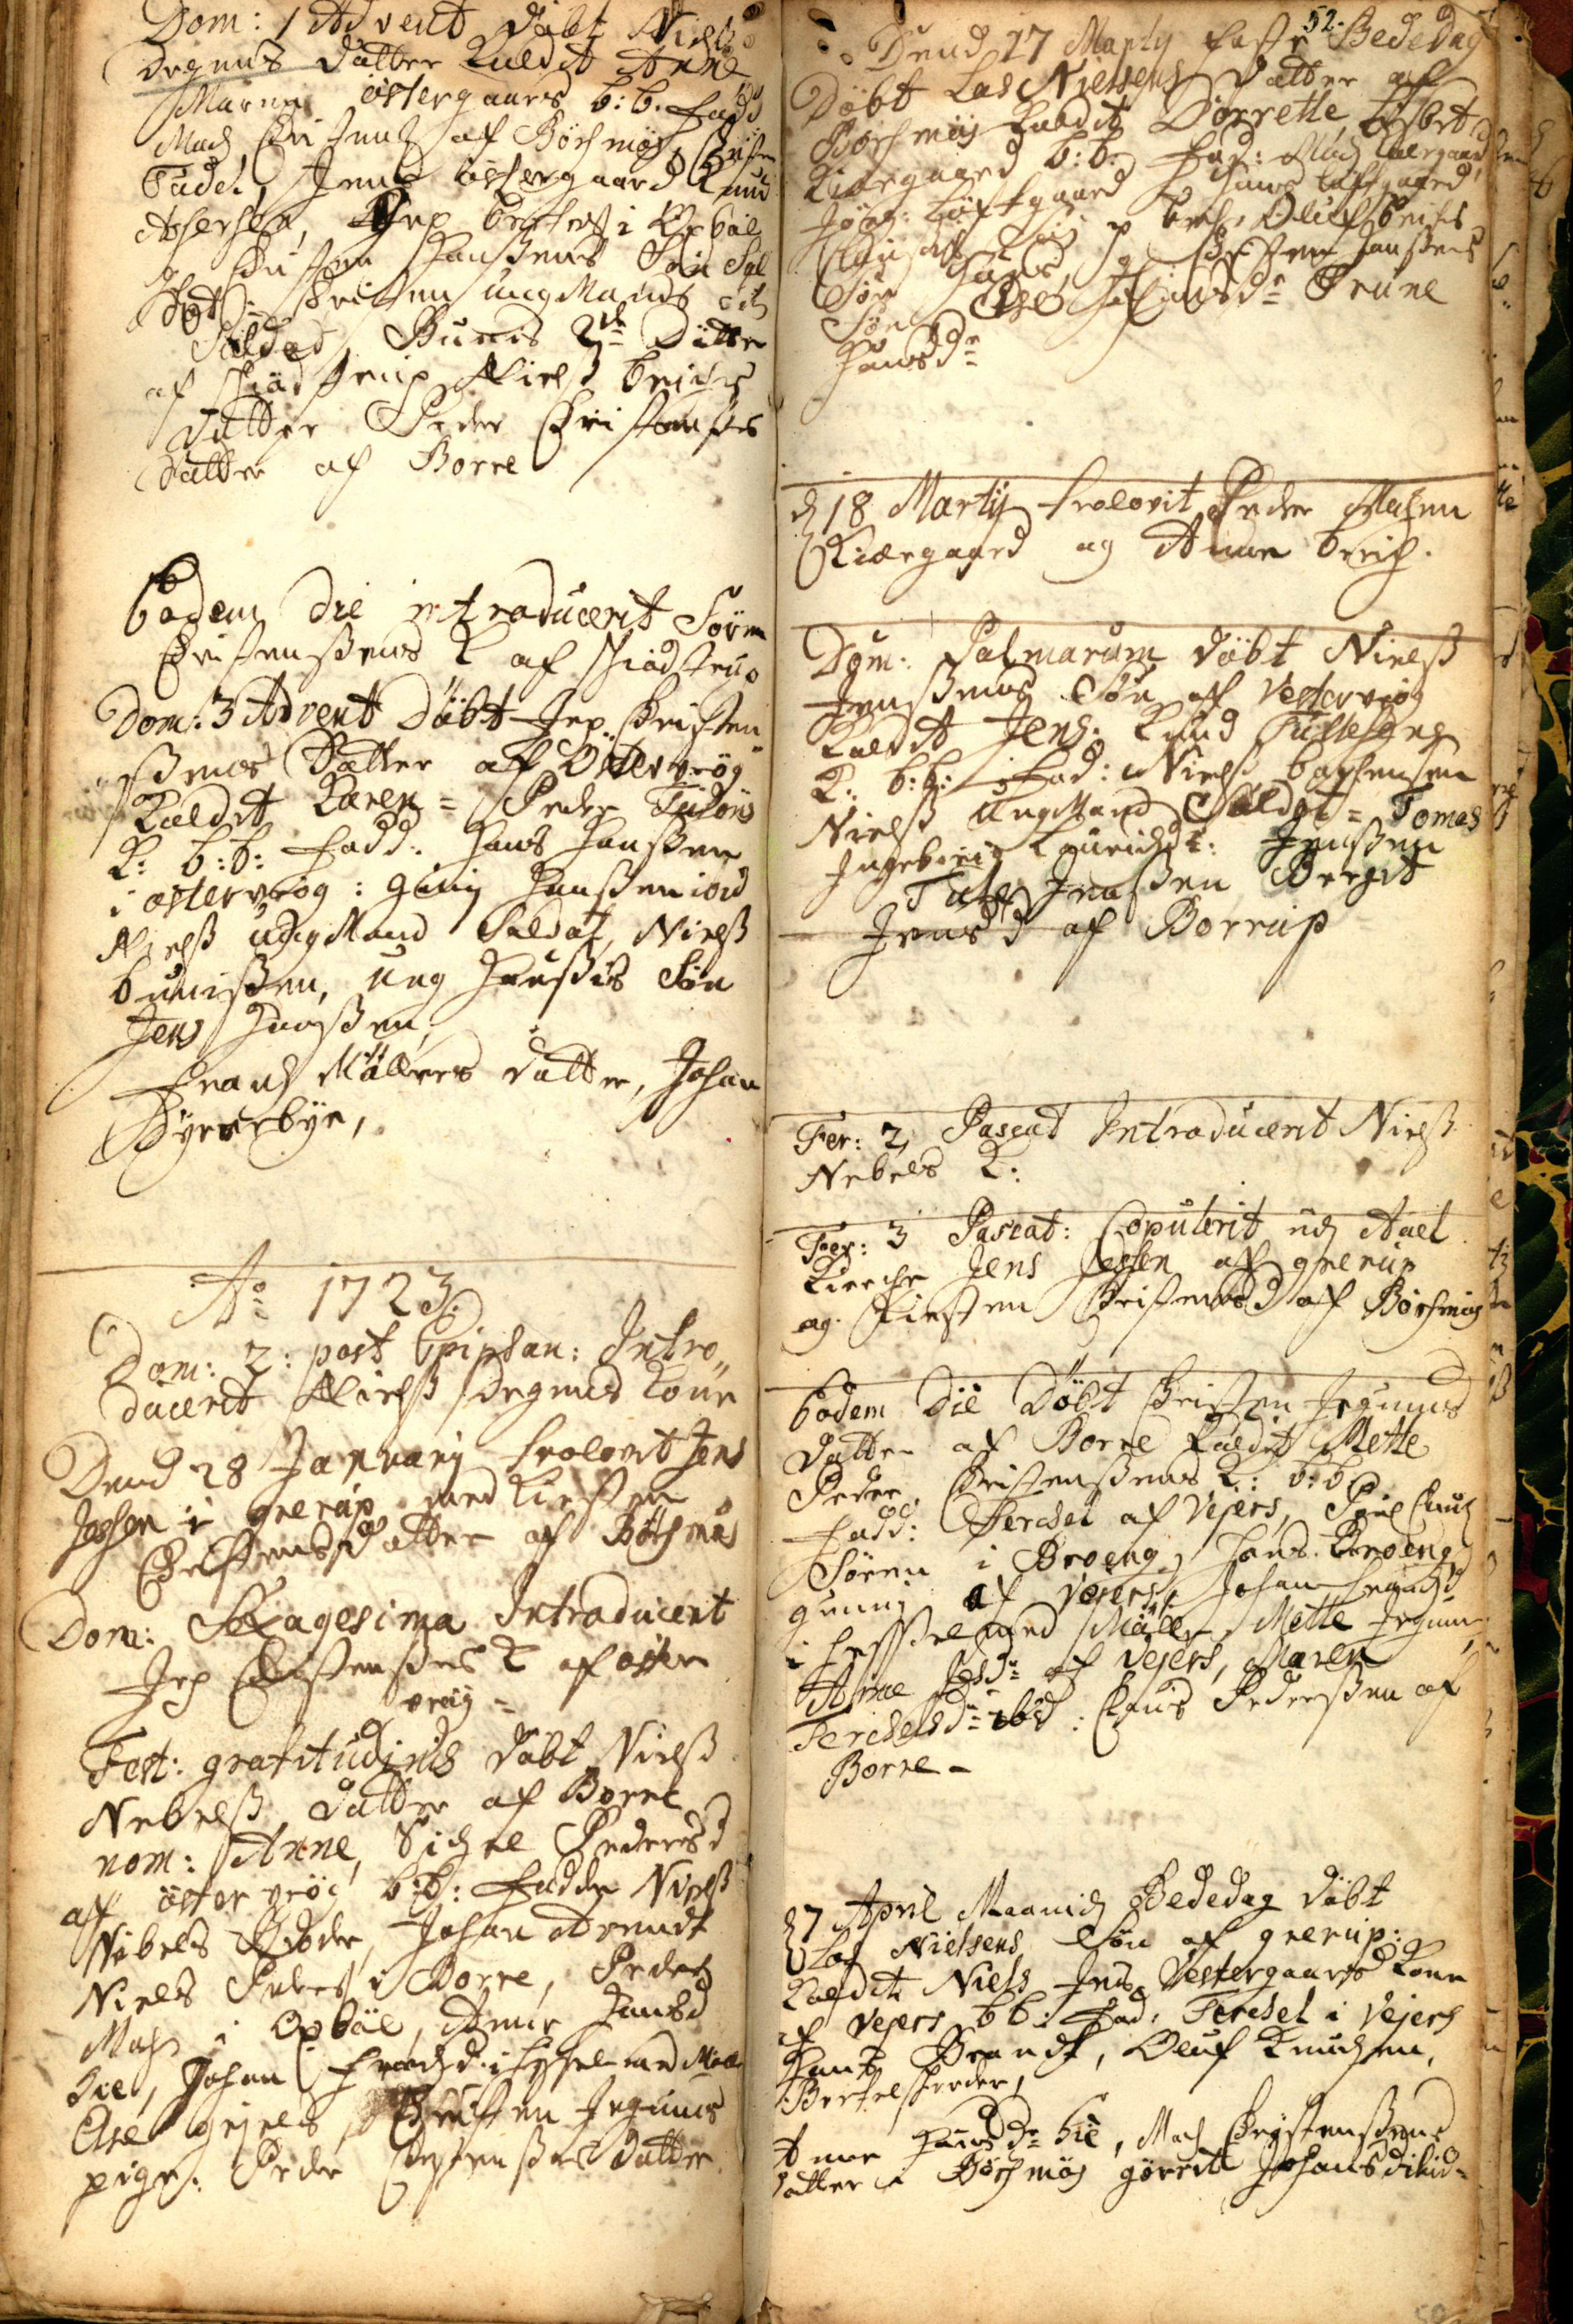Dom: 1 Advent døbt Niels
Degens Datter kaldit Anne
Maren Østergaars b:b: Fadd
Madz Christens af Børsmøs, Christen
Tudel, Jens Østergaard Knud
Assersen, Jep Bertelsen i Oxbøl
gl Christen Hansens Søn Sol
dat= Christen ung Mands dito
Soldat Bunis 2de Døttre
af Schiødstrup, Niels Brichis
Datter Peder Christensens
Datter af Borre
Eodem Die introducerit Søren
Christensens K af Schiødstrup
Dom: 3 Advent Døbt Jep Christen
sens Datter af Østervrøg
kaldit Karen= Peder Tusøns
K: b:b: Fadd: Hans Hansen
i Østrvrøg: Gunj Hansen ibid
Niels ung Mand Soldat, Niels
Bunisen, ung Hansis Søn
Jens Hansen
Frandz Møllers Datter, Johan
Dyrrebye,
Ao 1723.
Dom: 2: post Epiphan: Intro¬
ducerit Niels Degens Kone
Dend 28 Januarij trolovet Jens
Jessen i Grerup med Kirsten
Christensdatter af Børsmøs
Dom: Sexagesima Introducerit
Jep Christensens K af Øster
vrøg=
Fest: Gratitudinis døbt Niels
Nebels Datter af Borre
nom: Anne, Sidzel Pedersd
af Østervrøg b:b: Faddere Niels
Nebels Broder, Johan Arendt
Niels Peders i Borre, Peder
Mads i Oxbøl, Anne Hansd
##, Johanne Frandzd i Hisselmed Mølle
Else Gejels, Christen Jegums
pige: Peder Christensens Datter
52
50
Dend 17 Martij Faste Bededag
Døbt Las Nielsens Datter af
Børsmøs kaldit Dorrette, Lisbet
Kiærgaard b:b: Fad: Madz Kiærgaard
Jørg: Løftgaard Hans Løftgaard
Clauses Søn p Brich, Oluf Brichis
Søn Hans, gl Christen Hansens
Søn Else Johansdr Triine
Hansdr
D 18 Martij trolovit Peder Madzen
Kiærgaard og Anne Brich.
Dom: Palmarum døbt Niels
Jensens Søn af Vestervrøg
kaldit Jens. Knud Tullesens
K: b:b: Fad: Niels Bachensen
Niels ung Mand Soldat= Tomas
Ingeborig Lauridzdr Jensen##
Tule Jensen Bergit
Jensd af Borrup
Fer: 2 Pascat Introducerit Niels
Nebels K:
Fer: 3 Pascat: Copulerit udi Aael
Kirche Jens Jessen af Grerup
og Kirsten Christensd af Børsmøs
Eodem Die Døbt Christen Jegums
Datter af Borre kaldit Mette
Peder Christensens K: b:b:
Fadd: Therchel af Vejers, Poul Claus
Søren i Broeng, Hans Broeng
Gunnj af Vejers, Johanne Frandzdr
i Hisselmed Mølle, Mette Jegum,
Anne Jesdr af Vejers, Maren
Terchelsdr ibid: Claus Pedersen af
Borre
D 7 April Maanedz Bededag døbt
Las Nielsens Søn af Grerup:
kaldit Niels, Jes Vestergaars Kone
af Vejers bb: Fad: Terchel i Vejers,
Hans Brandt, Oluf Knudzen
Bertel Schreder,
Anne Hansdr hic##, Madz Christensens
Datter i Børsmøs Gørrit Johansd ibid:
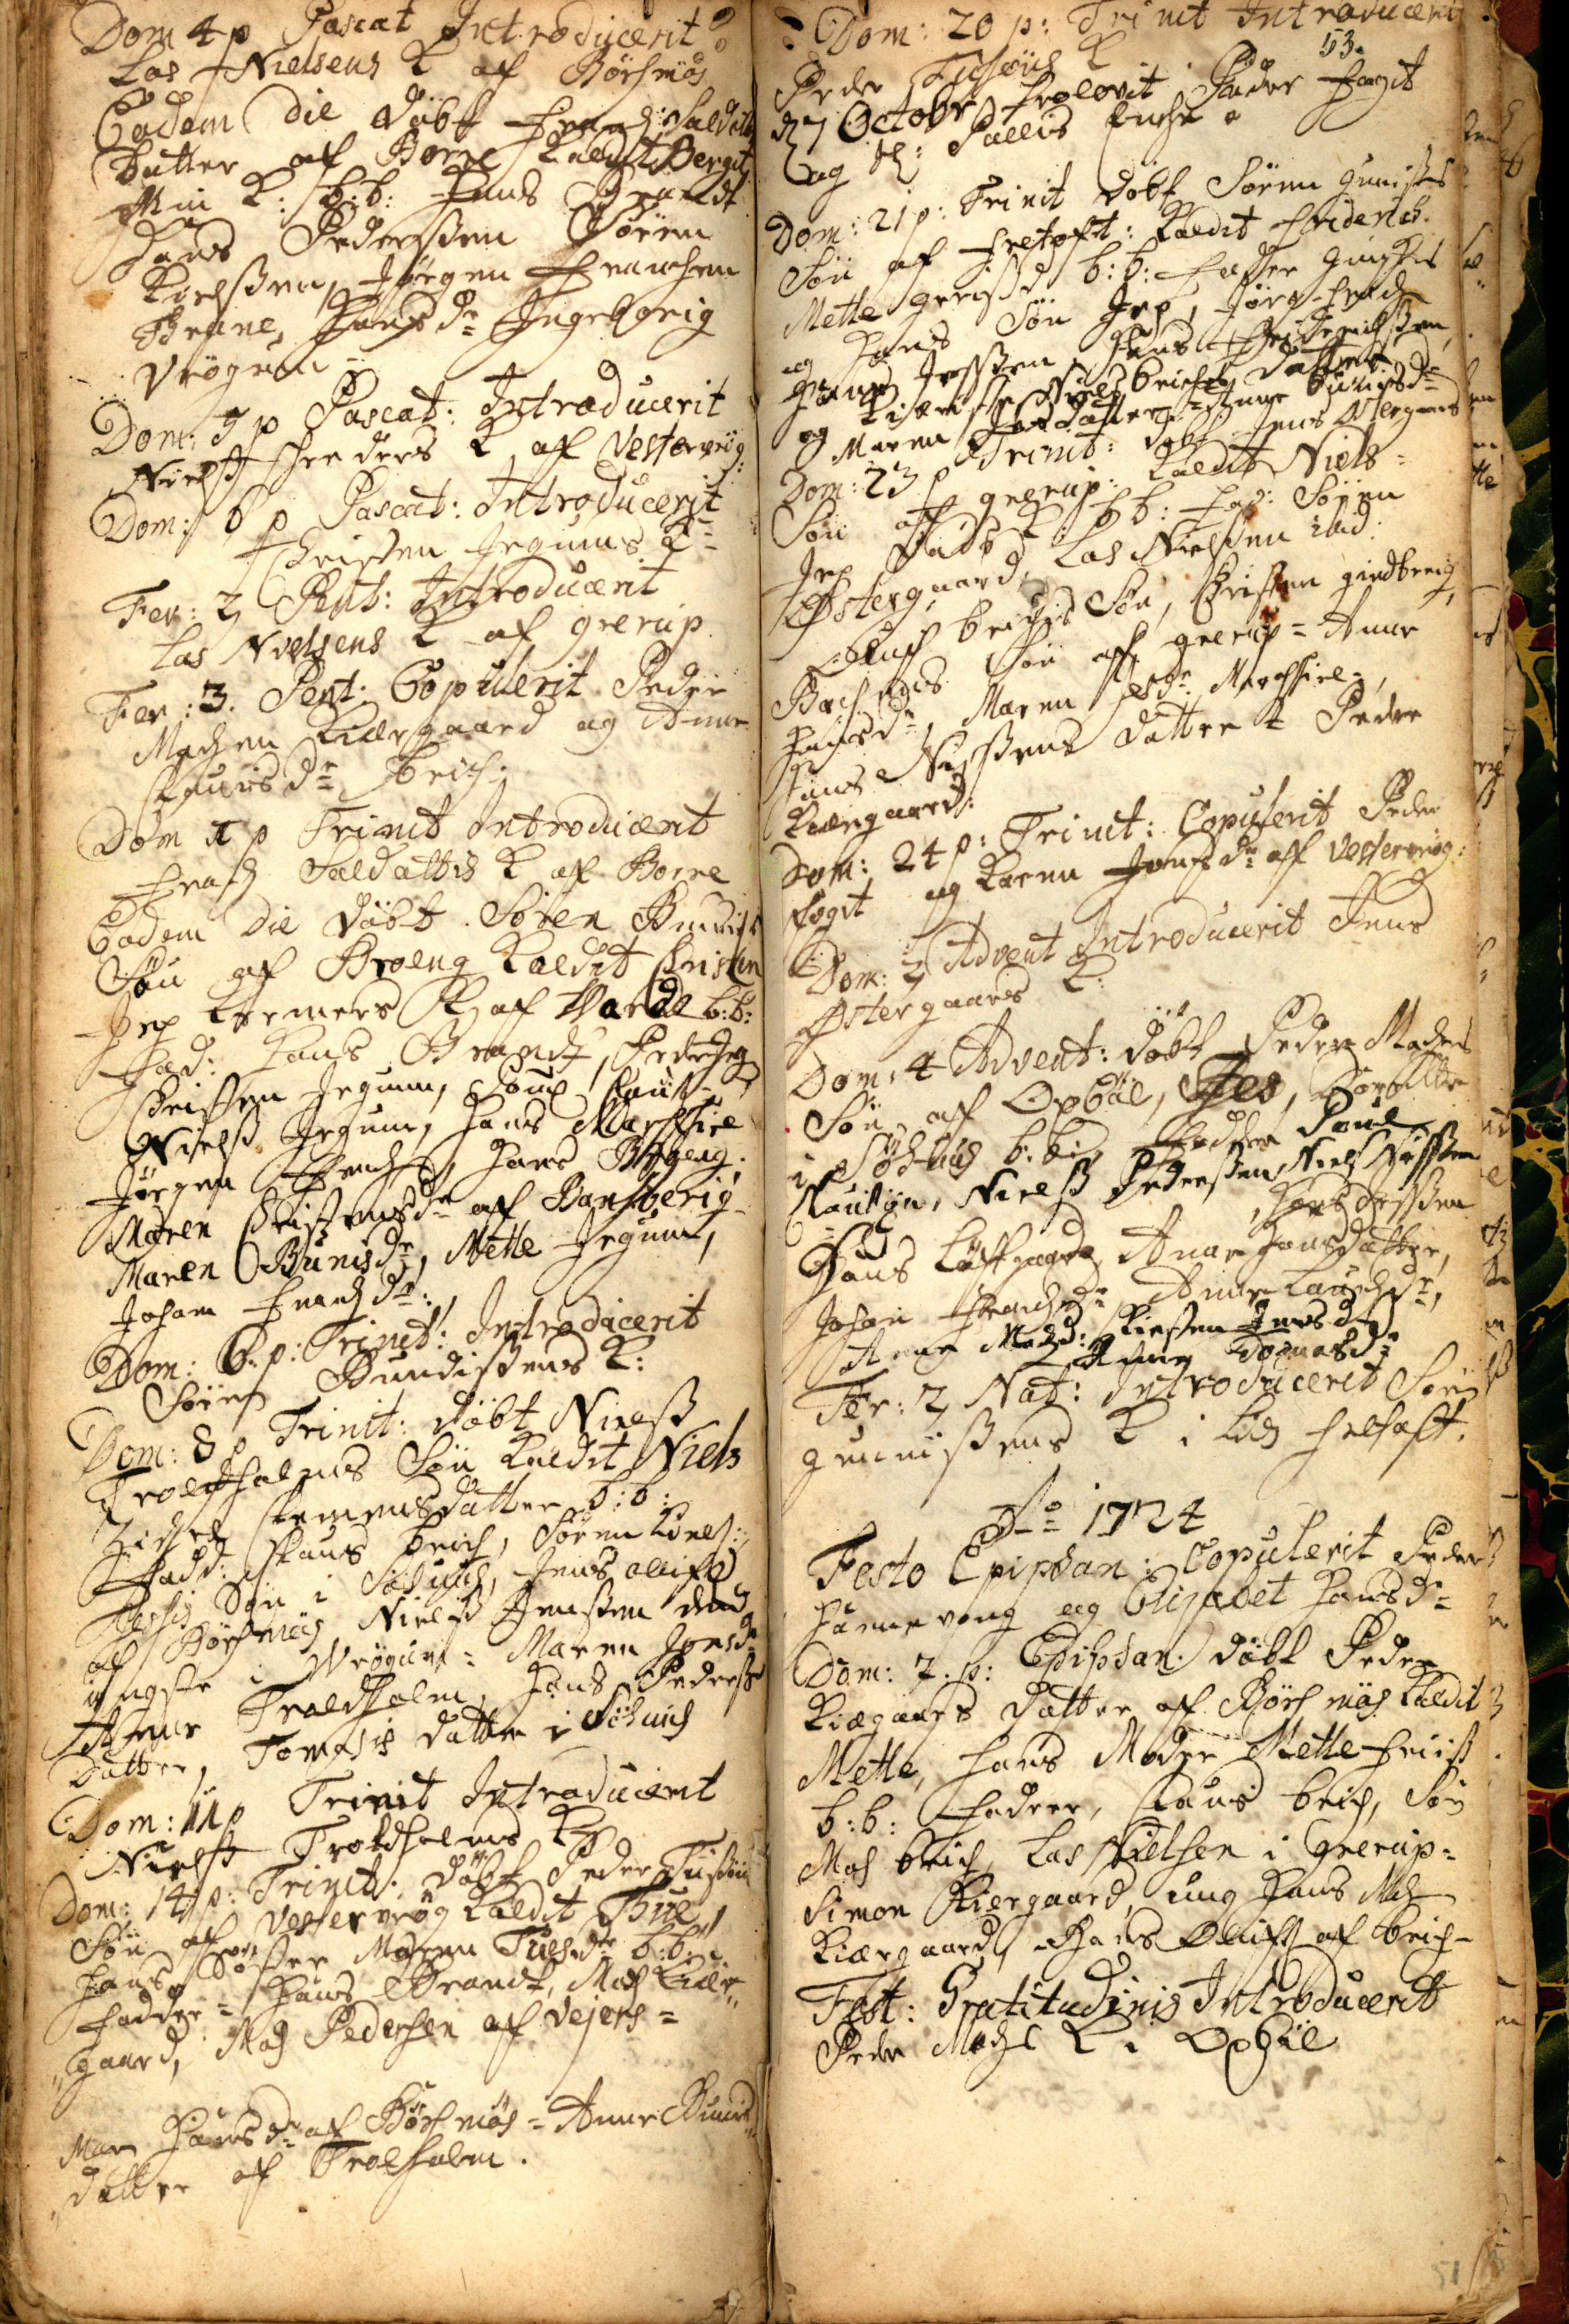Dom 4 p Pascat Introducerit
Las Nielsens K af Børsmøs
Eodem Die døbt Farndz Soldats
Datter af Borre kaldit Bergit
Min K: b:b: Hans Brandt
Hans Pedersen Søren
Kielsen, Jørgen Frandzen
Thriine Hansdr Ingeborig
Vrøgum
Dom: 5 p Pascat: Introducerit
Niels Schreders K af Vestervrøg
Dom: 6 p Pascat: Introducerit
Christen Jegums K=
Fer: 2 Pent: Introducerit
Las Nielsens K af Grerup
Fer: 3. Pent: Copulerit Peder
Madzen Kiærgaard og Anne
Clausdr Brich:
Dom 1 p Trinit Introducerit
Frandz Soldattis K af Borre
Eodem Die døbt Søren Bunnisens
Søn af Broeng kaldit Christen
Jep Kremers K af Warde b:b:
Fad: Hans Brandt, Peder Jeg
Christen Jegum, Poul Claus=
Niels Jegum, Hans Marchschiel
Jørgen Christens, Hans Broeng
Maren Christensdr af Bansberig
Maren Bunisdr, Mette Jegum,
Johanne Frandzdr:
Dom: 6: p: Trinit: Introducerit
Søren Bundisens K:
Dom: 8 p Trinit: døbt Niels
Troldholms Søn kaldit Niels
Zidzel Clemensdatter b:b:
Fadd: Claus Brich, Søren Kiels:
Jessis Søn i Søhuus, Jens Olufs
af Børsmos Niels Jensen dend
yngste i Wrøgum: Maren Jonsdr
Anne Troldholm, Hans Peders
Datter Tomasis Datter i Søhuus
Dom: 11 p Trinit Introducerit
Niels Troldholms K
Dom: 14 p: Trinit: døbt Peder Tussøns
Søn af Vestervrøg kaldit Thue,
Hans Søster Maren Tuesdr b:b:
Fadder= Hans Brandt, Madz Kiær¬
gaard, Madz Pedersen af Vejers=
Maren Hansdr af Børsmøs= Anne Bundis¬
datter af Troldholm.
53
51
Dom: 20 p:Trinit Introducerit
Peder Tusøns K.
D 7 Octobr trolovet Peder Foget
og Sl Pallis Enche ##
Dom: 21 p: Trinit døbt Søren Gunisens
Søn af Fretoft: kaldit Friderich.
Mette Greisd b:b:Fadder Gunj H##
og hans Søn Jep, Jørgen Frandz
Hans Jessen, Hans Friderichsen
og Kiæreste, Niels Brichis Datter.
Maren Jesdatter= Anne Tullisdr
Dom: 23 p Trinit: døbt Jens Østergaars
Søn af Grerup: kaldit Niels=
Jep Vads K: bb: Fad: Søren
Østergaard, Las Nielsen ibid:
Oluf Brichis Søn, Christen Giedberig
Bachens Søn af Grerup= Anne
Hansdr, Maren Jesdr Marchschiel=
Hans Nissens Datter=  Peder
Kiærgaard:
Dom: 24 p: Trinit: Copulerit Peder
Fogit og Karen Jonsdr af Vestervrøg:
Dom: 2 Advent Introducerit Jens
Østergaars K:
Dom: 4 Advent: døbt Peder Madzens
Søn af Oxbøl, Jes, Dorrette
i Søhuus b:b: Faddere Poul
Clausøn, Niels Pedersen, Niels Nissen
Hans Jessen
Hans Løftgaard, Anne Hansdatter,
Johan Frandzdr Anne Lauridzdr,
Anne Madzd: Kirsten Jensd
Anne Tomasdr
Fer: .2 Nat: Introducerit Søren
Gunnisens K i Lid Fretoft
Ao 1724
Festo Epiphan: Copulerit Peder
Hannevang og Elisabet Hansdr
Dom: 2: p: Epiphan. døbt Peder
Kiærgaars Datter af Børsmøs kaldit
Mette, hans Moder Mette Friis
b:b: Fadere Claus Brich, Søn
Madz Brich, Las Nielsen i Grerup=
Simon Kiærgaard, ung Hans Madz##
Kiærgaard, Hans Olufsen af Brich-
Fest: Gratutidinis Introducerit
Peder Madzs K i Oxbøl